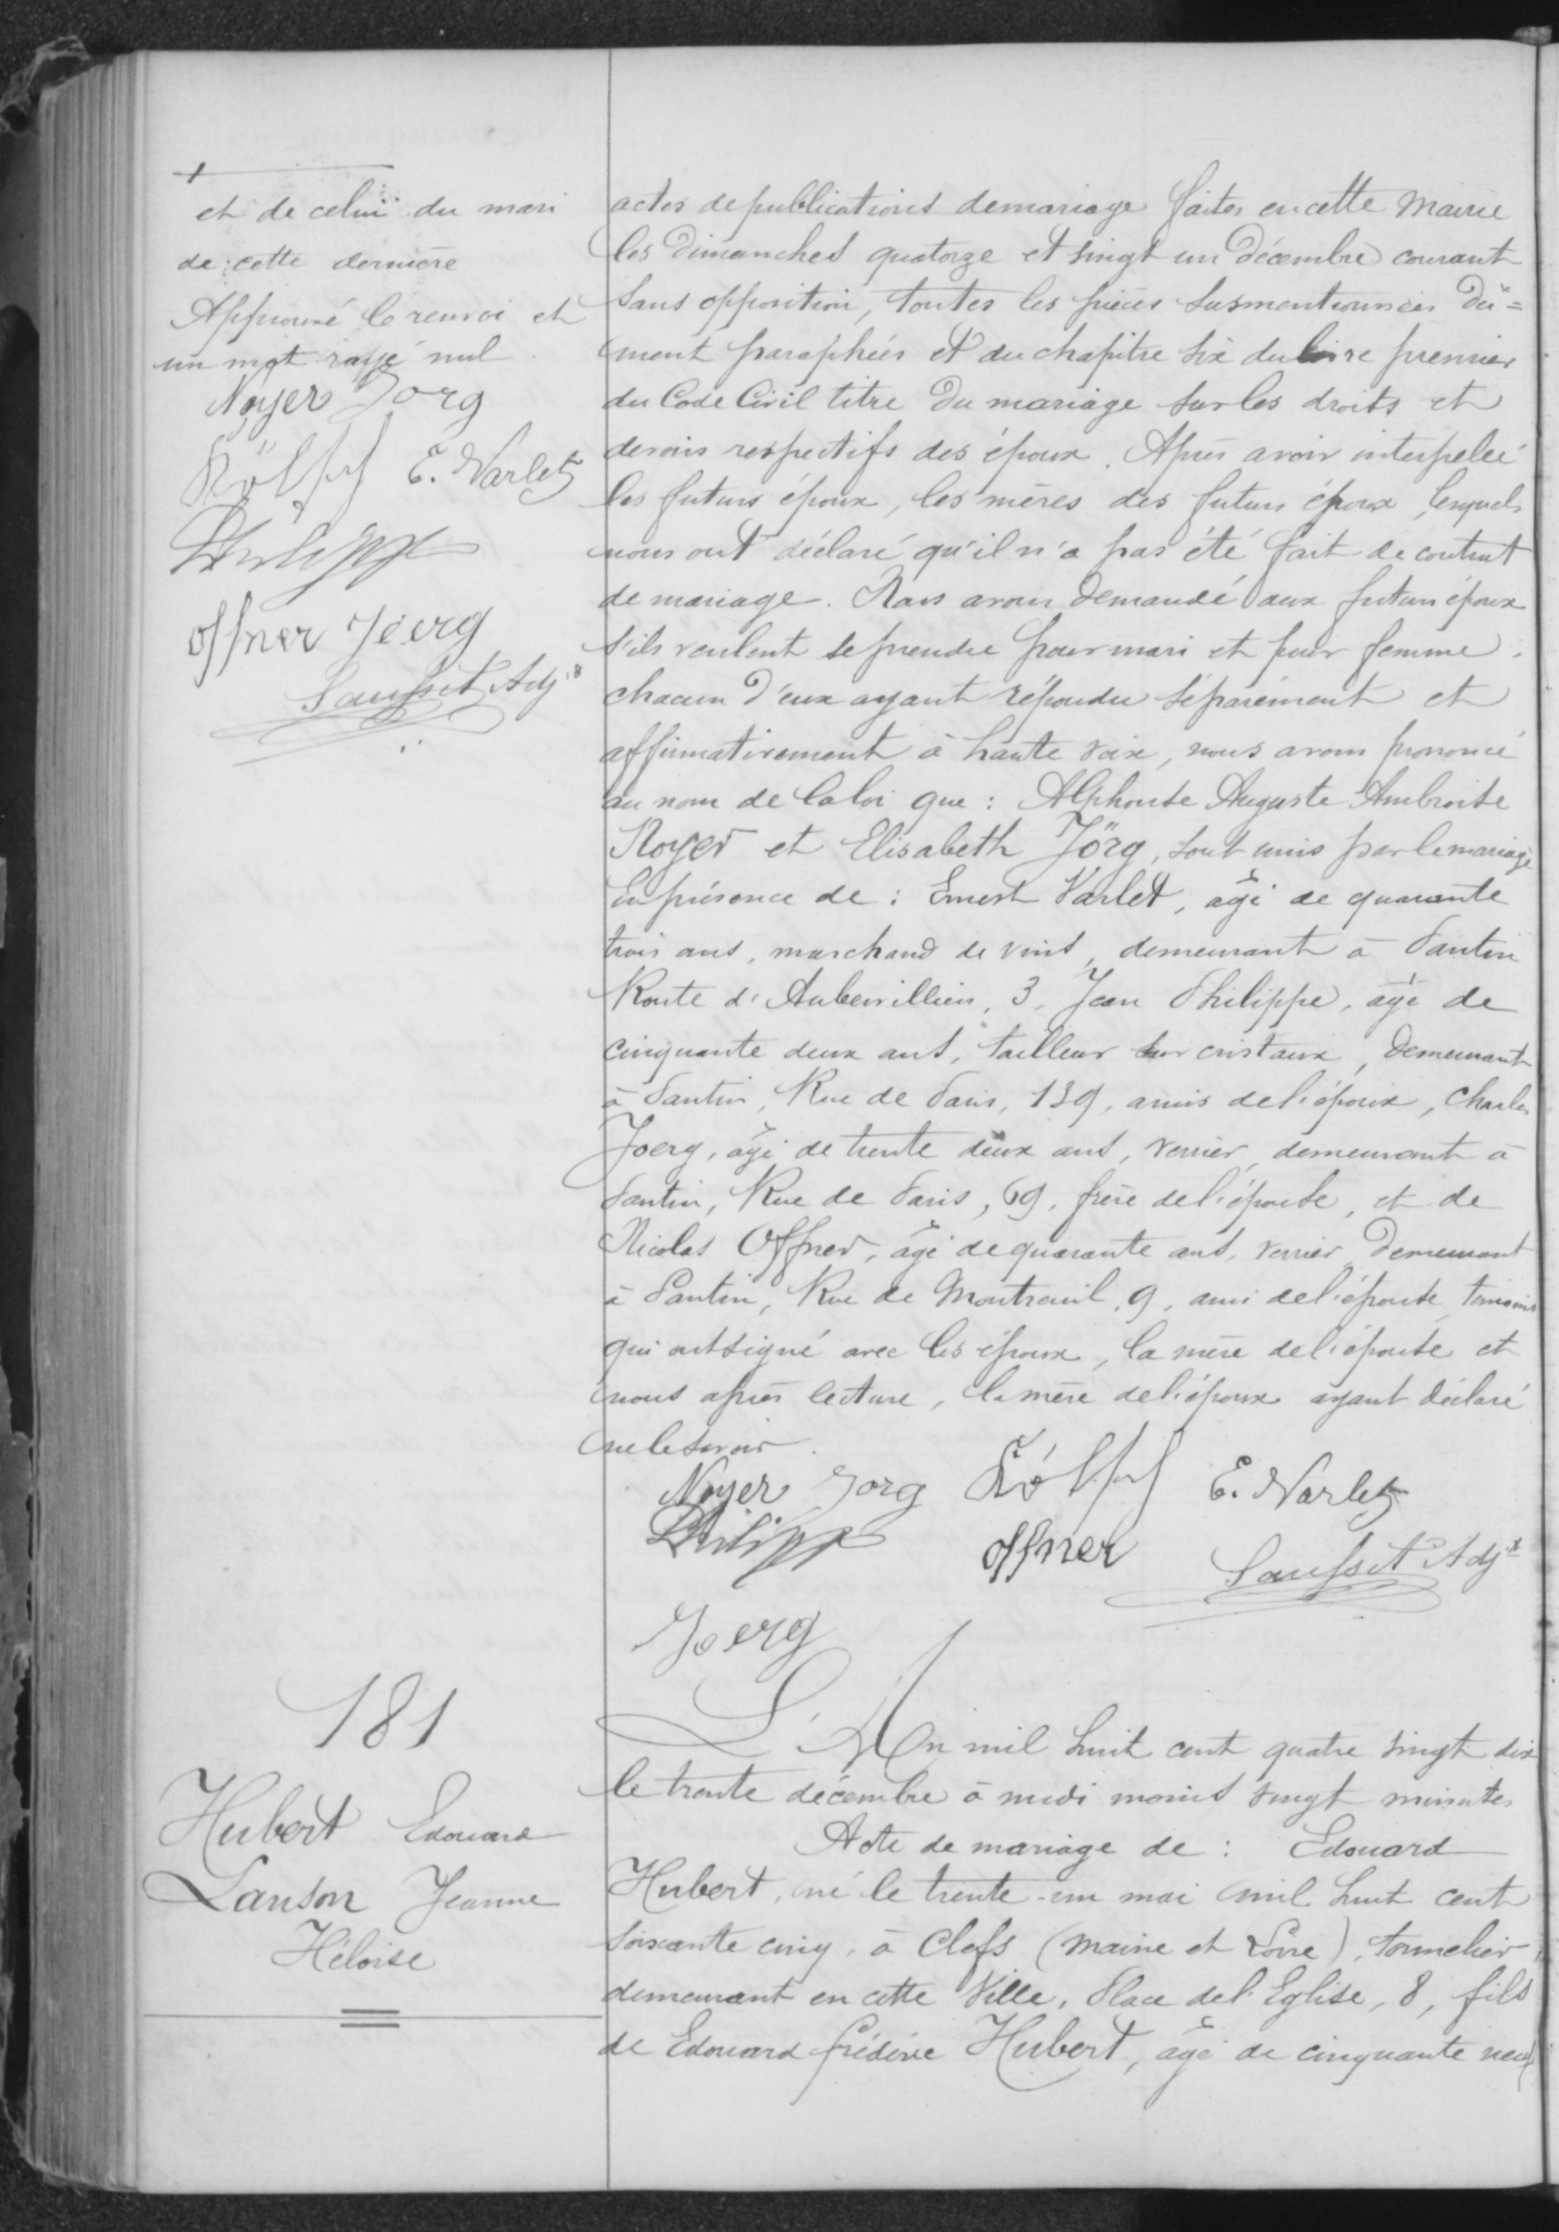actes de publications demariage faites en cette mairie
les dimanche quatorze et vingt un décembre courant
sans opposition, toutes les pièces susmentionnées dû
ment paraphées et du chapitre six du livre premier
du Code Civil titre du mariage sur les droits et
devoirs respectifs des époux. Après avoir interpelé
les futurs époux, les mères des futurs époux, lesquels
nous ont déclaré qu'il n'a pas été fait de contrat
de mariage. Nous avons demandé aux futurs époux
s'ils veulent se prendre pour mari et pour femme
chacun d'eux ayant répondu separément et
affirmativement à haute voix, nous avons prononcé
au nom de la loi que: Alphonse Auguste Ambroise
Royer et Elisabeth Yörg, sont unis par le mariage.
En présence de: Ernest Varlet, âgé de quarante
trois ans, marchand de vins, demeurant à Pantin
Route d'Aubervilliers, 3, Jean Philippe, âgé de
cinquante deux ans, tailleur sur cristaux, demeurant
à Pantin, Rue de Paris, 139, amis del'épouse, Charles
Joerg, âgé de trente deux ans, verrier, demeurant à
Pantin, Rue de Paris, 69, frère del'épouse, et de
Nicolas Offrer, âgé de quarante ans, verrier, demeurant
à Pantin, Rue de Montreuil, 9, ami del'épouse témoins
qui ont signé avec les époux, la mère del'épouse et
nous après lecture, la mère del'époux ayant déclaré
ne le savoir.
181
Hubert Edouart
Lanson Jeanne
Héloïse
L'An mil huit cent quatre vingt dix
le trente décembre à midi moins vingt minutes
Acte de mariage de: Edouard
Hubert, né le trente-un mai mil huit cent
soixante cinq, à Clefs (Maine et Loire), tournelier
demeurant en cette Ville, Place del'Eglise, 8, fils
de Edouard frédéric Hubert, âgé de cinquante neuf
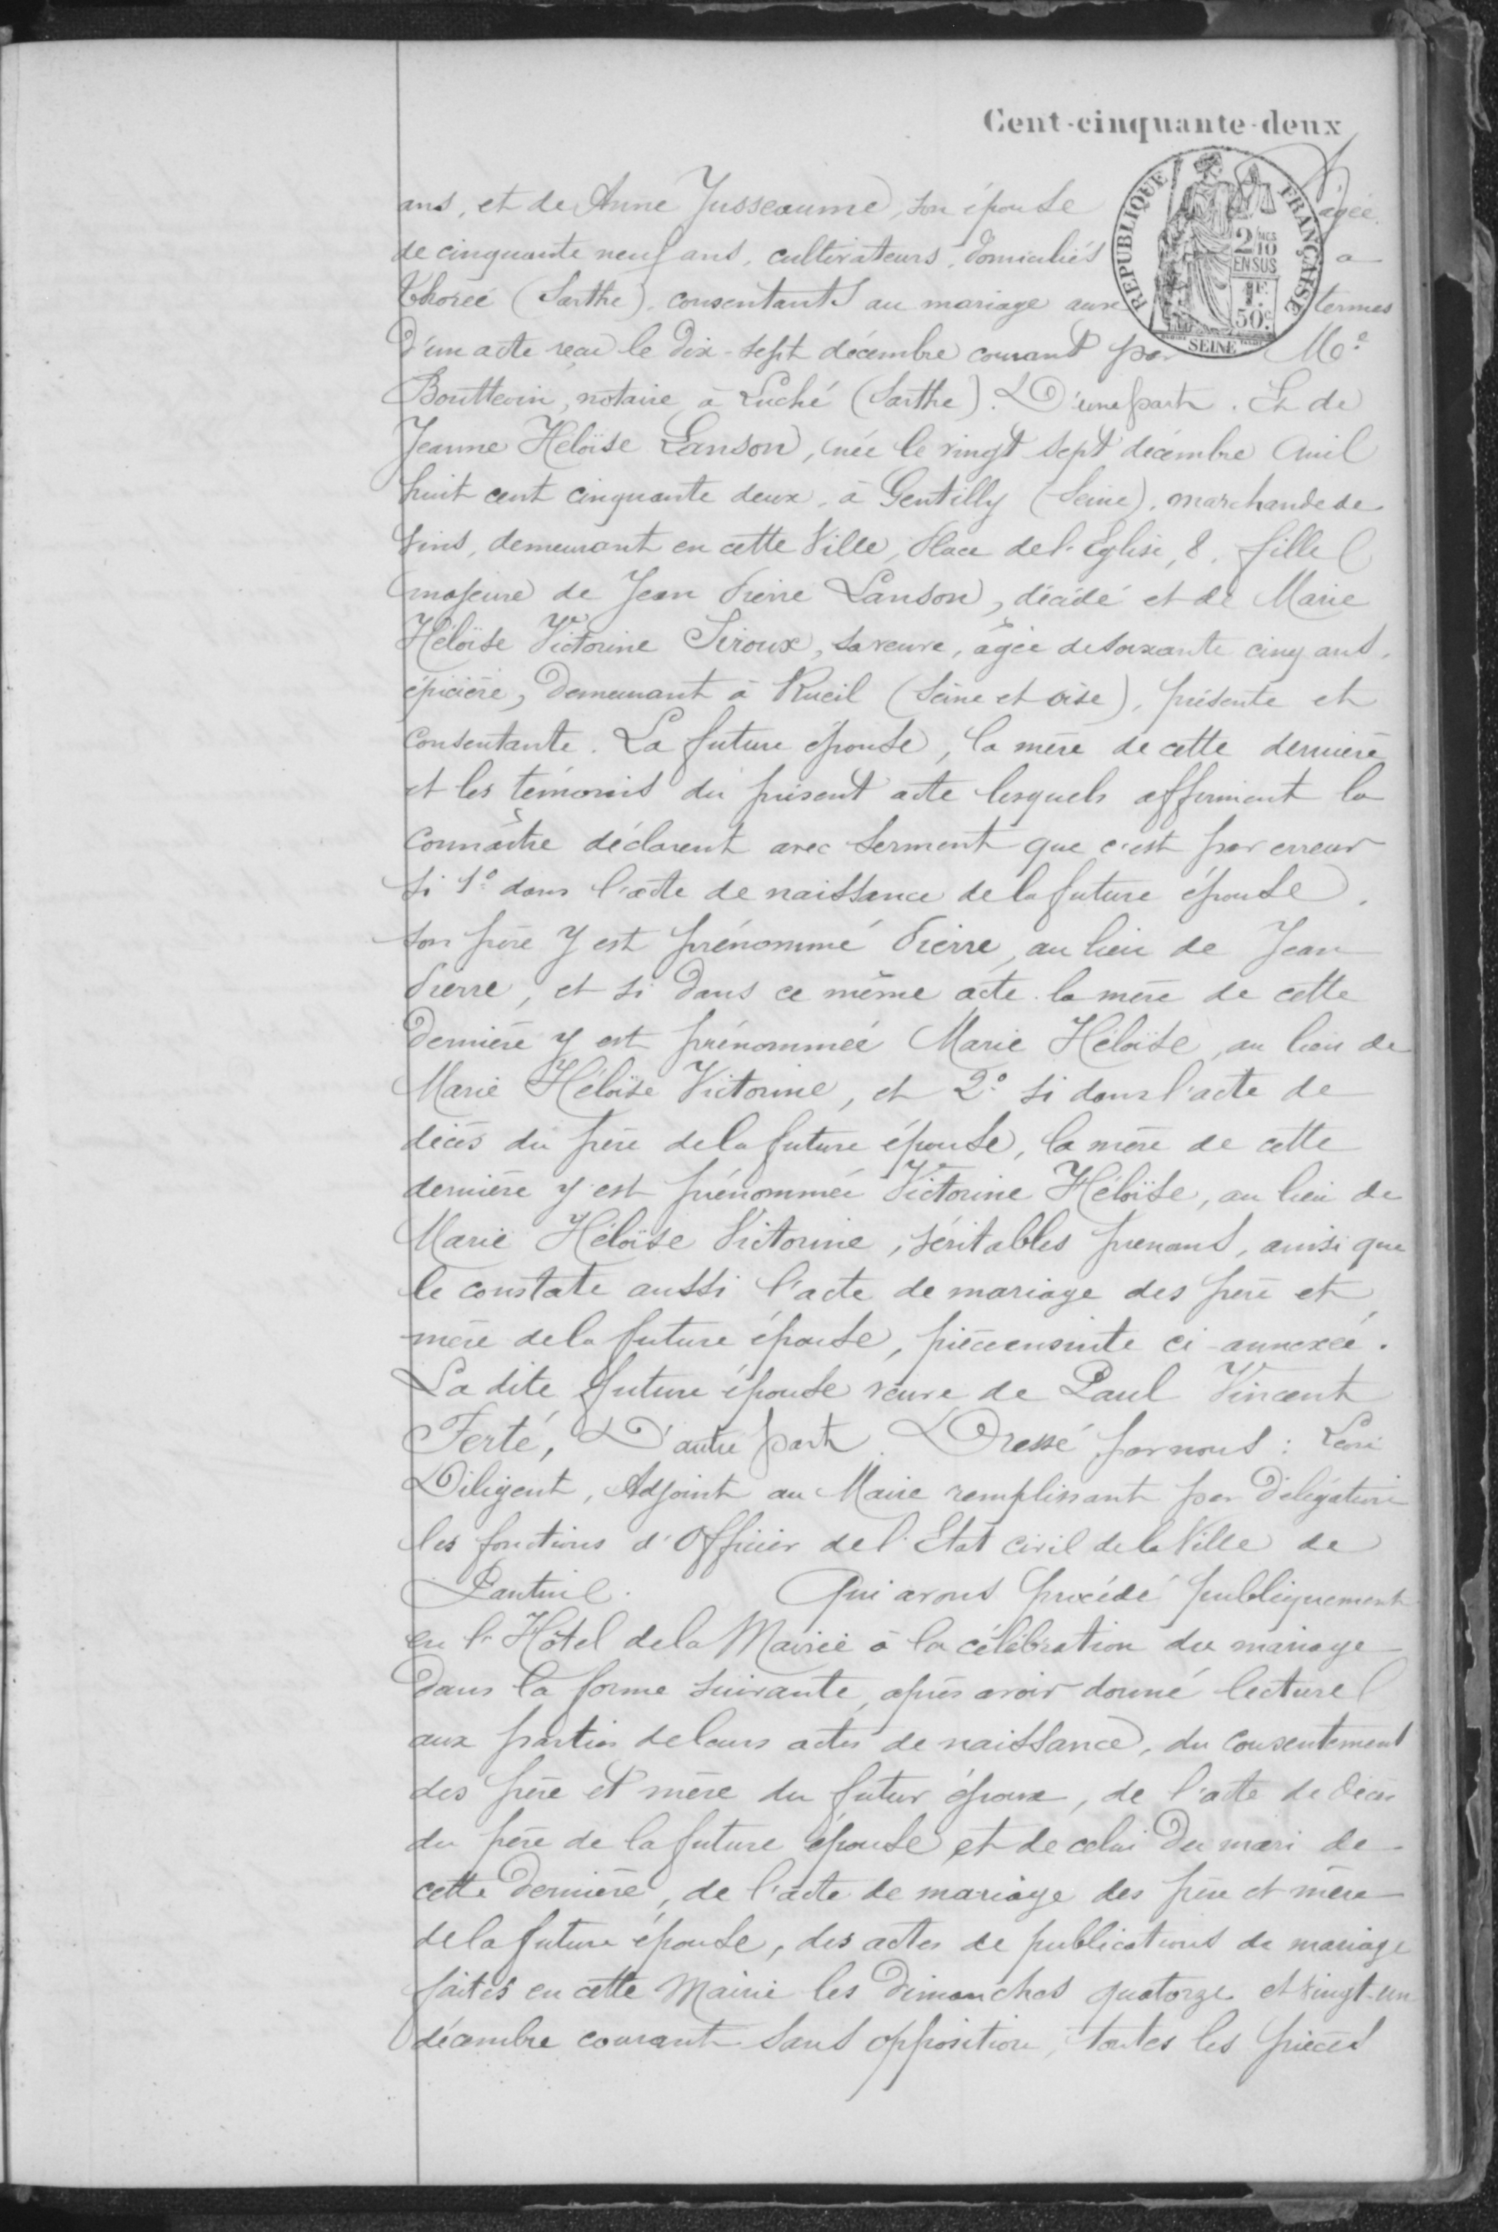ans, et de Anne Yuzzeaume, son épouse agée
de cinquante neuf ans, cultivateurs, domicilié a
Thorée (Sarthe), consentants au mariage aux termes
d'un acte reçu le dix-sept décembre courant par M°
Boutterin, notaire à Suché (Sarthe) D'une part. Et de
Jeanne Héloïse Lason, née le vingt-sept décembre mil
huit cent cinquante deux, à Gentilly (Seine), marchande de
Vins, demeurant en cette Ville, Place del'Eglise, 8, fille
(majeure) de Jean Pierre Lason, décédé et de Marie
Héloïse Victorine Siroux, sa veuve, âgée de soixante cinq ans,
épicière, demeurant à Reuil (Seine et Oise), présent et
consentant. La future épouse, la mère de cette dernière
et les témoins du présent acte lesquels affirment la
connaître déclarent avec Serment que c'est par erreur
si 1° dans l'acte de naissance de la future épouse
son père y est prénommé Pierre, au lieu de Jean
Pierre, et si dans ce même acte la mère de cette
dernière y est prénommée Marie Héloïse au lieu de
Marie Héloïse Victorine, et 2° si dans l'acte de
décès du frère dela future épouse, la mère de cette
dernière y est prénommée Victorine Heloïse, au lieu de
Marie Héloïse Victorine, véritables prenoms, ainsi que
le constate aussi l'acte de mariage des père et
mère dela future épouse, pièceensuite ci-annexée.
La dite future épouse veuve de Paul Vincent
Ferte, D'autre part. Dressé par nous: Levi
Diligent, Adjoint au Maire remplissant par délégation
les fonctions d'Officier del'Etat Civil de la Ville de
Pantin. Qui avons procédé publiquement
en l'Hôtel dela Mairie à la célébration du mariage
dans la forme suivante, après avoir donné lecture
aux parties de leurs actes de naissance, du consentement
des frère et mère du futur époux, de l'acte de décès
du père de la future épouse et de celui du mari de
cette dernière, de l'acte de mariage des père et mère
dela future épouse, des actes de publications de mariage
faites en cette Mairie les dimanches quatorze et vingt-un
décembre courant sans opposition toutes les pièces